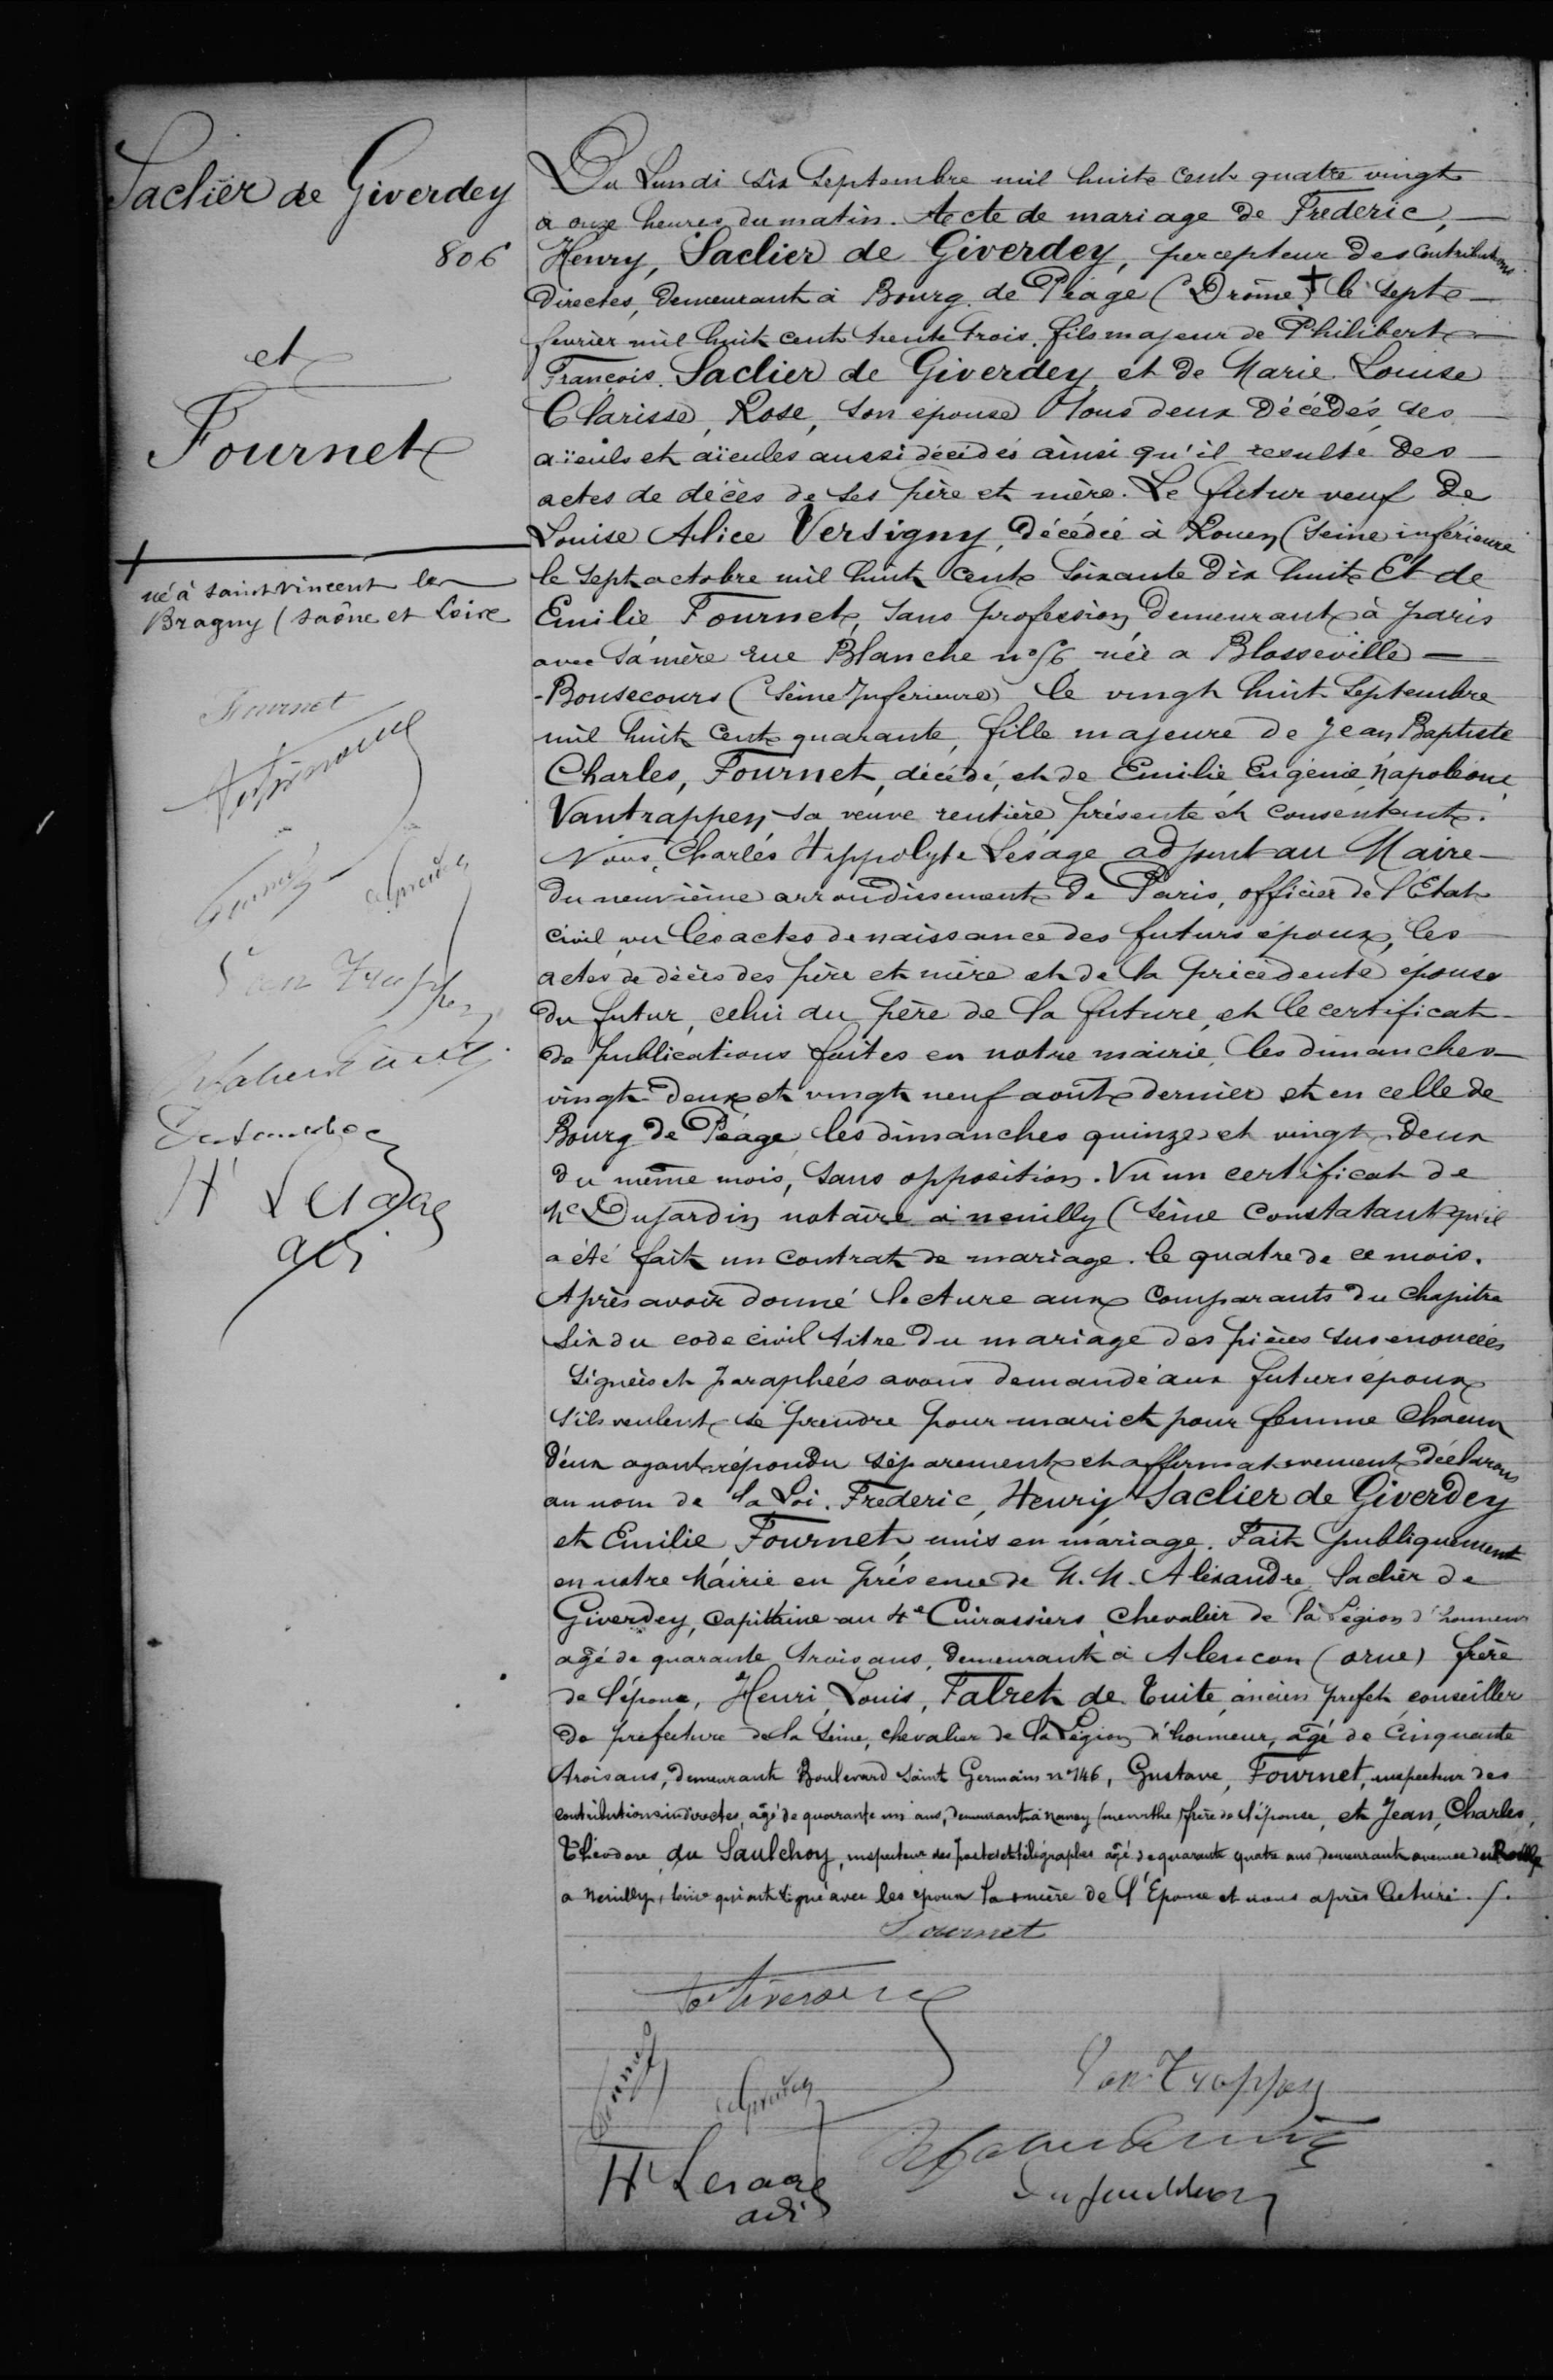Saclier de Giverdey
806
et
Fournete
Le Lundi si septembre mil huit cent quatre vingt
à onze heures du matin. Acte de mariage de Frederic,
Henry, Saclier de Giverdey, percepteur des contributions
directes, demeurant à Bourg de Prage (Drôme) né à Saint-Vincent le Bragny (Saône et Loire) le sept
fevrier mil huit cent trente trois, fils majeur de Philibert
François Saclier de Giverdey et de Marie Louise
Clarisse, Rose, son épouse tous deux décédés ses
aïeuls et aïeules aussi décédés ainsi qu'il resulte des
actes de décès de ses père et mère. Le futur veuf de
Louise Alice Versigny, décédé à Rouen (Seine inférieure
le sept octobre mil huit cent soixante dix huit Et de
Emilie Fournet, sas profession demeurant à paris
avec sa mère rue Blanche n°56 née à Blosseville-
-Bonsecours (Seine Inferieure) le vingt huit septembre
mil huit cent quarante, fille majeure de Jean Baptiste
Charles, Fournet, décéé, et de Emilie Eugénie, Napoléone
Vantrappen sa veuve rentière présente et consentante.
Nous, Charles Hippolyte Lesage adjoint au Maire
du neuvième arrondissement de Paris, officier de l'Etat
civil du les actes de naissance des futurs époux les
actes de décès des père et mère et de la préscédente épouse
du futur, celui du père de la future, et le certificat
de publications faites en notre mairie les dimanches
vingt deux et vingt neuf août dernier et en celle de
Bourg de Péage les dimanches quinze et vingt deux
du même mois, sans opposition; Vu un certificat de
Me Dujardin notraire à neuilly (Seine constatant qu'il
a été fait un contrat de mariage le quatre de ce mois
Après avoir donné lecture aux comparants du chapitre
six du code civil titre du mariage des pièces sus enoncées
signées et paraphées avons demandé aux futurs époux
s'ils veulent se prendre pour mai et pour femme chacun
d'eux ayant répondu séparement et affirmativement déclarons
au nom de la Loi. Frederic, Henri Saclier de Giverdey
et Emilie Fournet, unis en mariage. Fait publiquement
en notre Mairie en présence de M.M Alexandre Saclier de
Giverdey, capitaine au 4e Cuirassiers chevalier de la Légion d'honneur
agé de quarante trois ans, demeurant à Alencon (Arue) frère
de l'époux, Henri, Louis, Falreh de Tuite, ancien prefet conseiller
de prefecture de la Seine, chevalier de la Légion d'honneur, âgé de cinquante
trois ans, demeurant Boulevard Saint Germain n°146, Gustave, Fournet, inspecteur des
contributions directes, âgé de quarante un ans, demeurant à Nancy (Meurthe) frère de l'épouse, et Jean, Charles
Théodore du Saulchoy, inspecteur de postes et télégraphes âgé de quarante quatre ans demeurant avenue du Roule
a Neuilly, témoins qui ont signé avec les époux la mère de l'Epoux et nous après lecture ./.
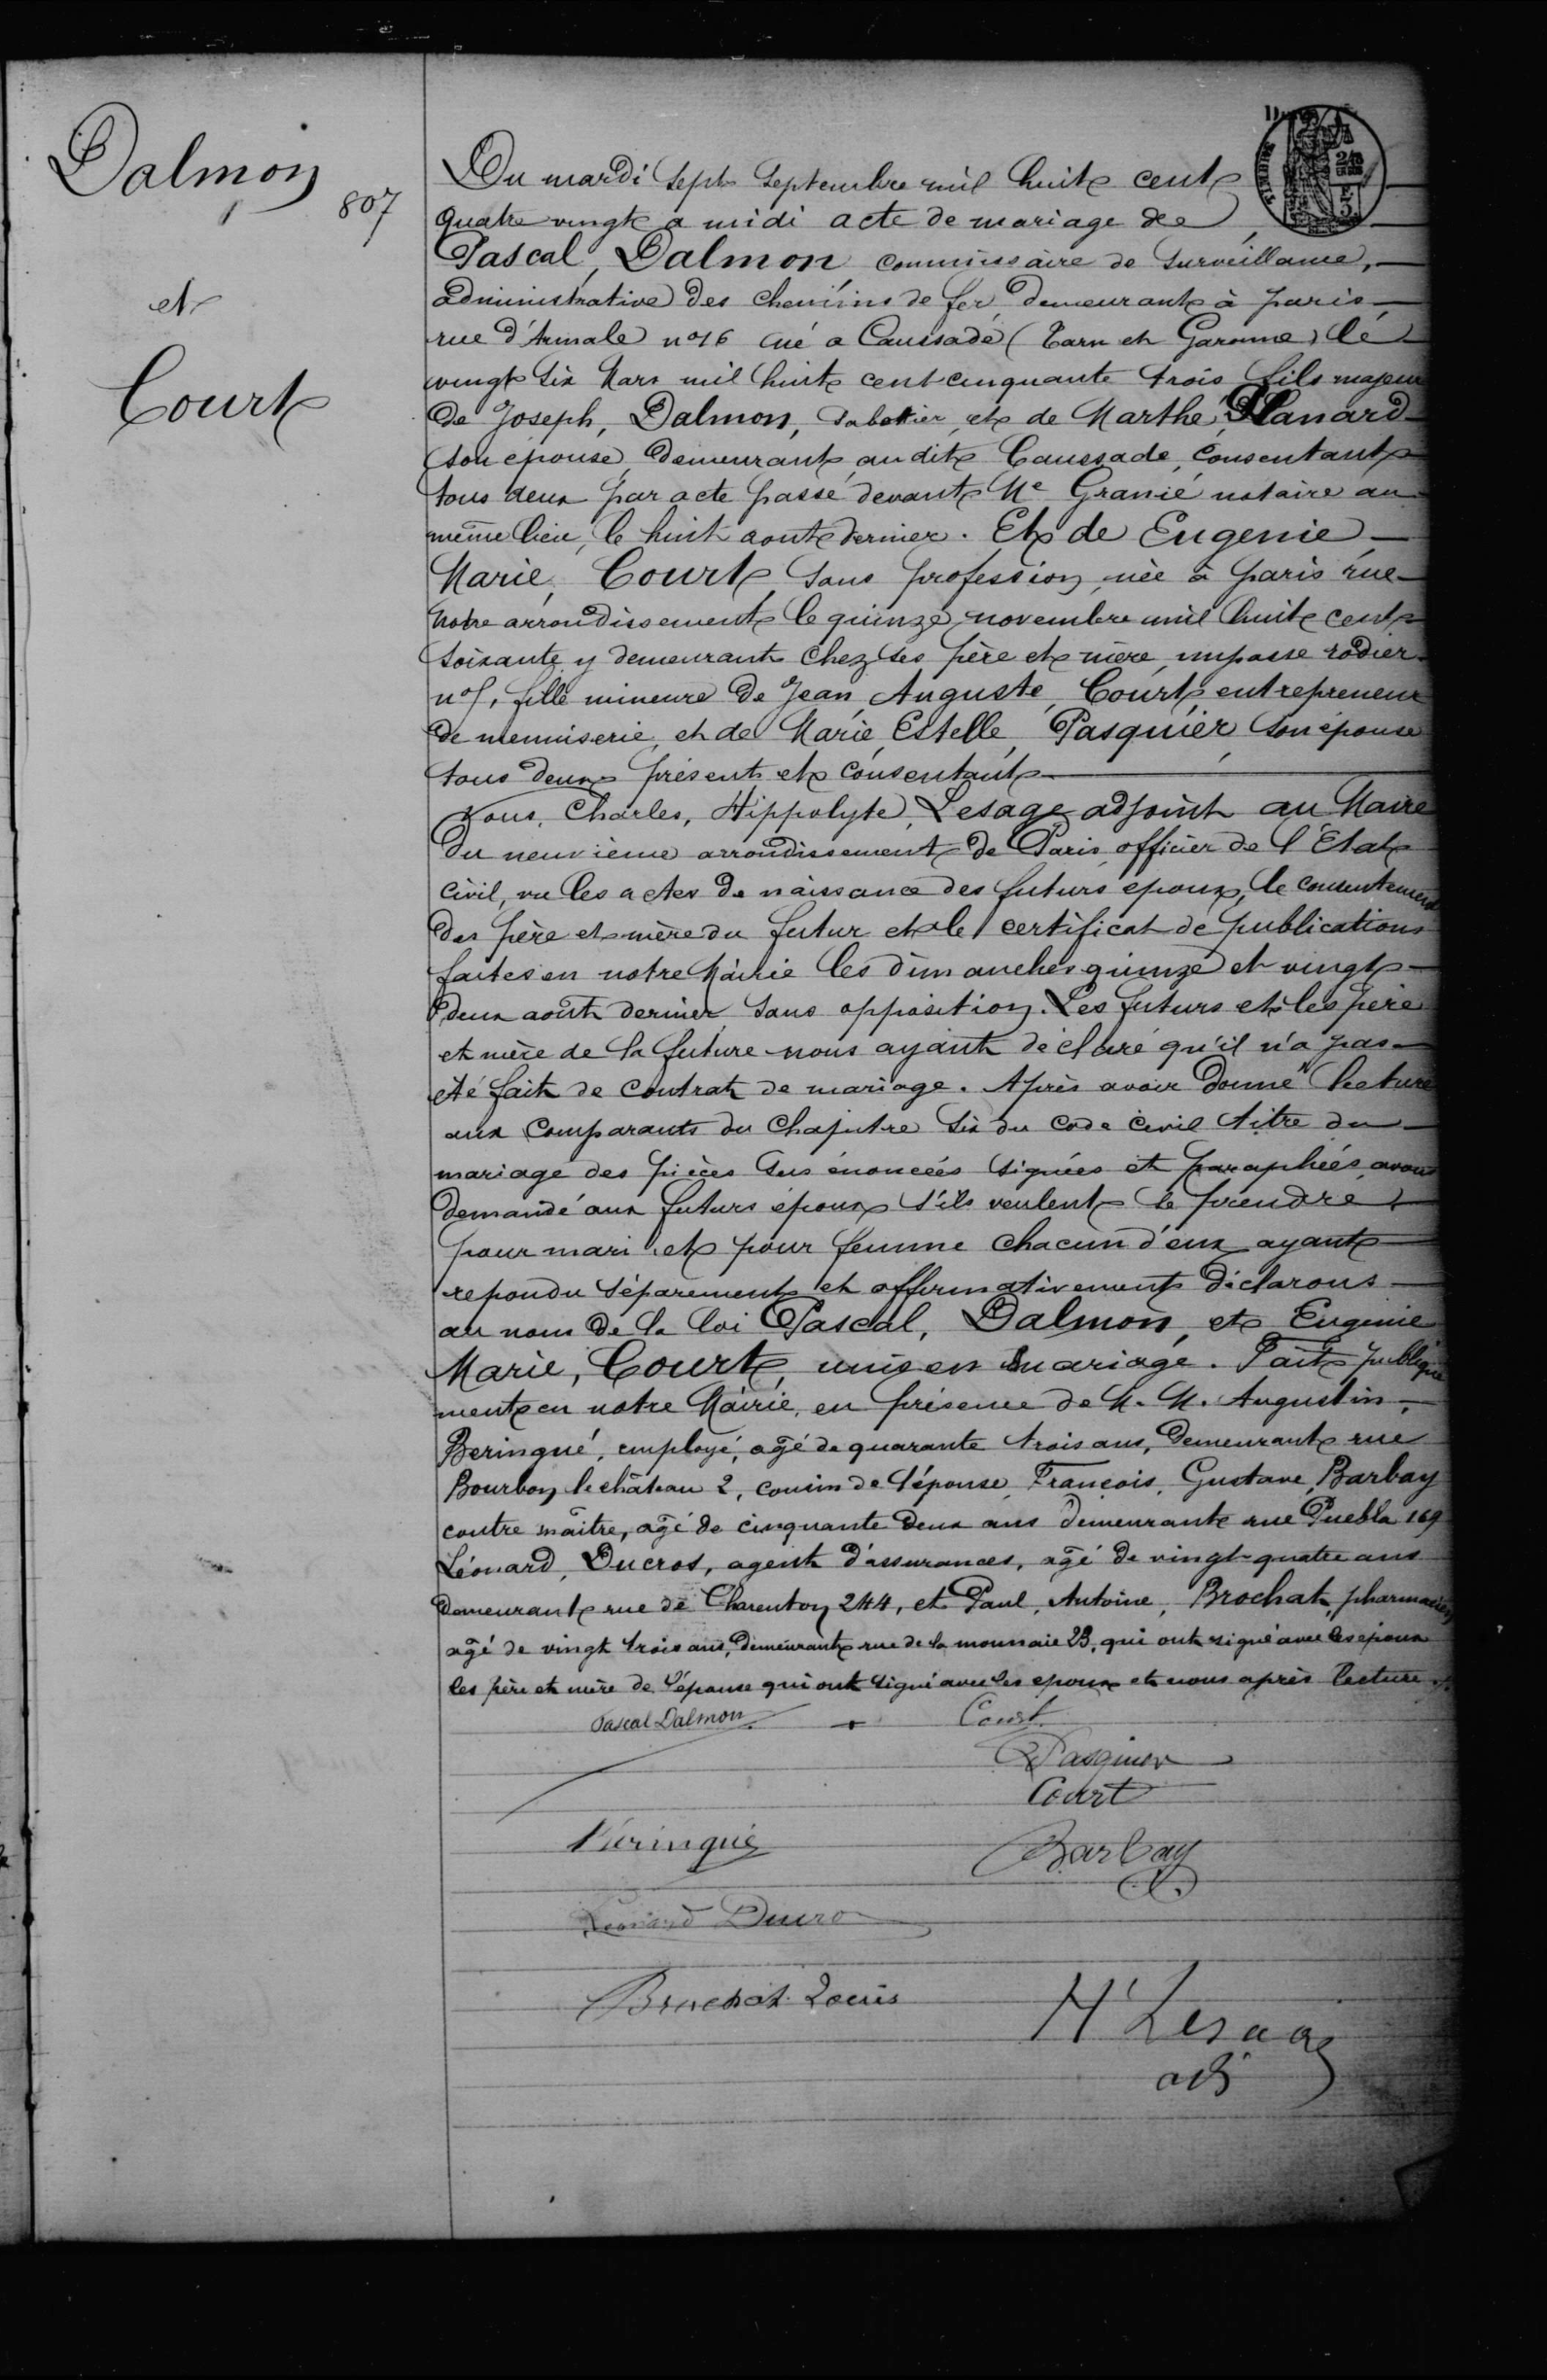Dalmon
807
et
Court
Du mardi sept septembre mil huit cent
quatre vingt a midi acte de mariage de
Pascal Dalmon commissaire de surveillance,
administrative des chemins de fer demeurant à paris
rue d'Aumale n°16 né à Caussade (Tarn et Garonne) le
vingt six Mars mil huite cent cinquante trois fils majeur
de Joseph, Dalmon, sabottier, et de Marthe Hanard
(son épouse) demeurant au dite Caussade, consentant
tous deux par acte passé devant Me Granié notaire au
même lieu, le huit aoute dernier. Et de Eugénie
Marie, Courte sans profession, née à paris rue
notre arrondissement le quinze novembre mil huite cent
soixante y demeurant chez ses père et mère, impasse rodier
n7, fille mineure de Jean Auguste Courte, entrepreneur
de menuiserie, et de Marie, Estelle Pasquier son épouse
tous deux présents et consentants
Vous, Charles, Hippolyte, Lesage adjoint au Maire
du neuvième arrondissement de Paris, officier de l'Etat
Civil, vu les actes de naissance des futurs epoux, le consentement
des père et mère du futur et le certificat de publication
faites en notre Mairie les dimanches quinze et vingt
deux août dernier sans opposition. Les futurs et les père
et mère de la future nous ayant déclaré qu'il n'a pas
été fait de contrat de mariage. Après avoir donné lecture
aux comparants des chapitre six du code civil titre du
mariage des pièces sus énoncées signées et paraphées avons
demandé aux futurs époux s'ils veulent se prendre
pour mari et pour femme chacun d'eux ayant
répondu séparement et affirmativement déclarons
au nom de la loi Pascal, Dalmon, et Eugenie
Marie, Courte, unis en mariage. Faite publique
ment en notre Mairie, en présence de M.M. Augustin
Beringué, employé, âge de quarante trois ans, demeurant rue
Bourbon, le château 2, cousin de l'épouse François Gustave, Barbay
contre maître, agé de cinquante deux ans demeurant rue Puebla 169
L2onard, Ducros, agent d'assurances, agé de vingt quatre ans
demeurant rue de Charenton, 244 et Paul, Antoine, Brochat, pharmacien
agé de vingt trois ans, demeurant rue de la monnaie 23, qui ont signé avec les époux
les père et mère de l'épouse qui ont signé avec les époux et nous après lecture